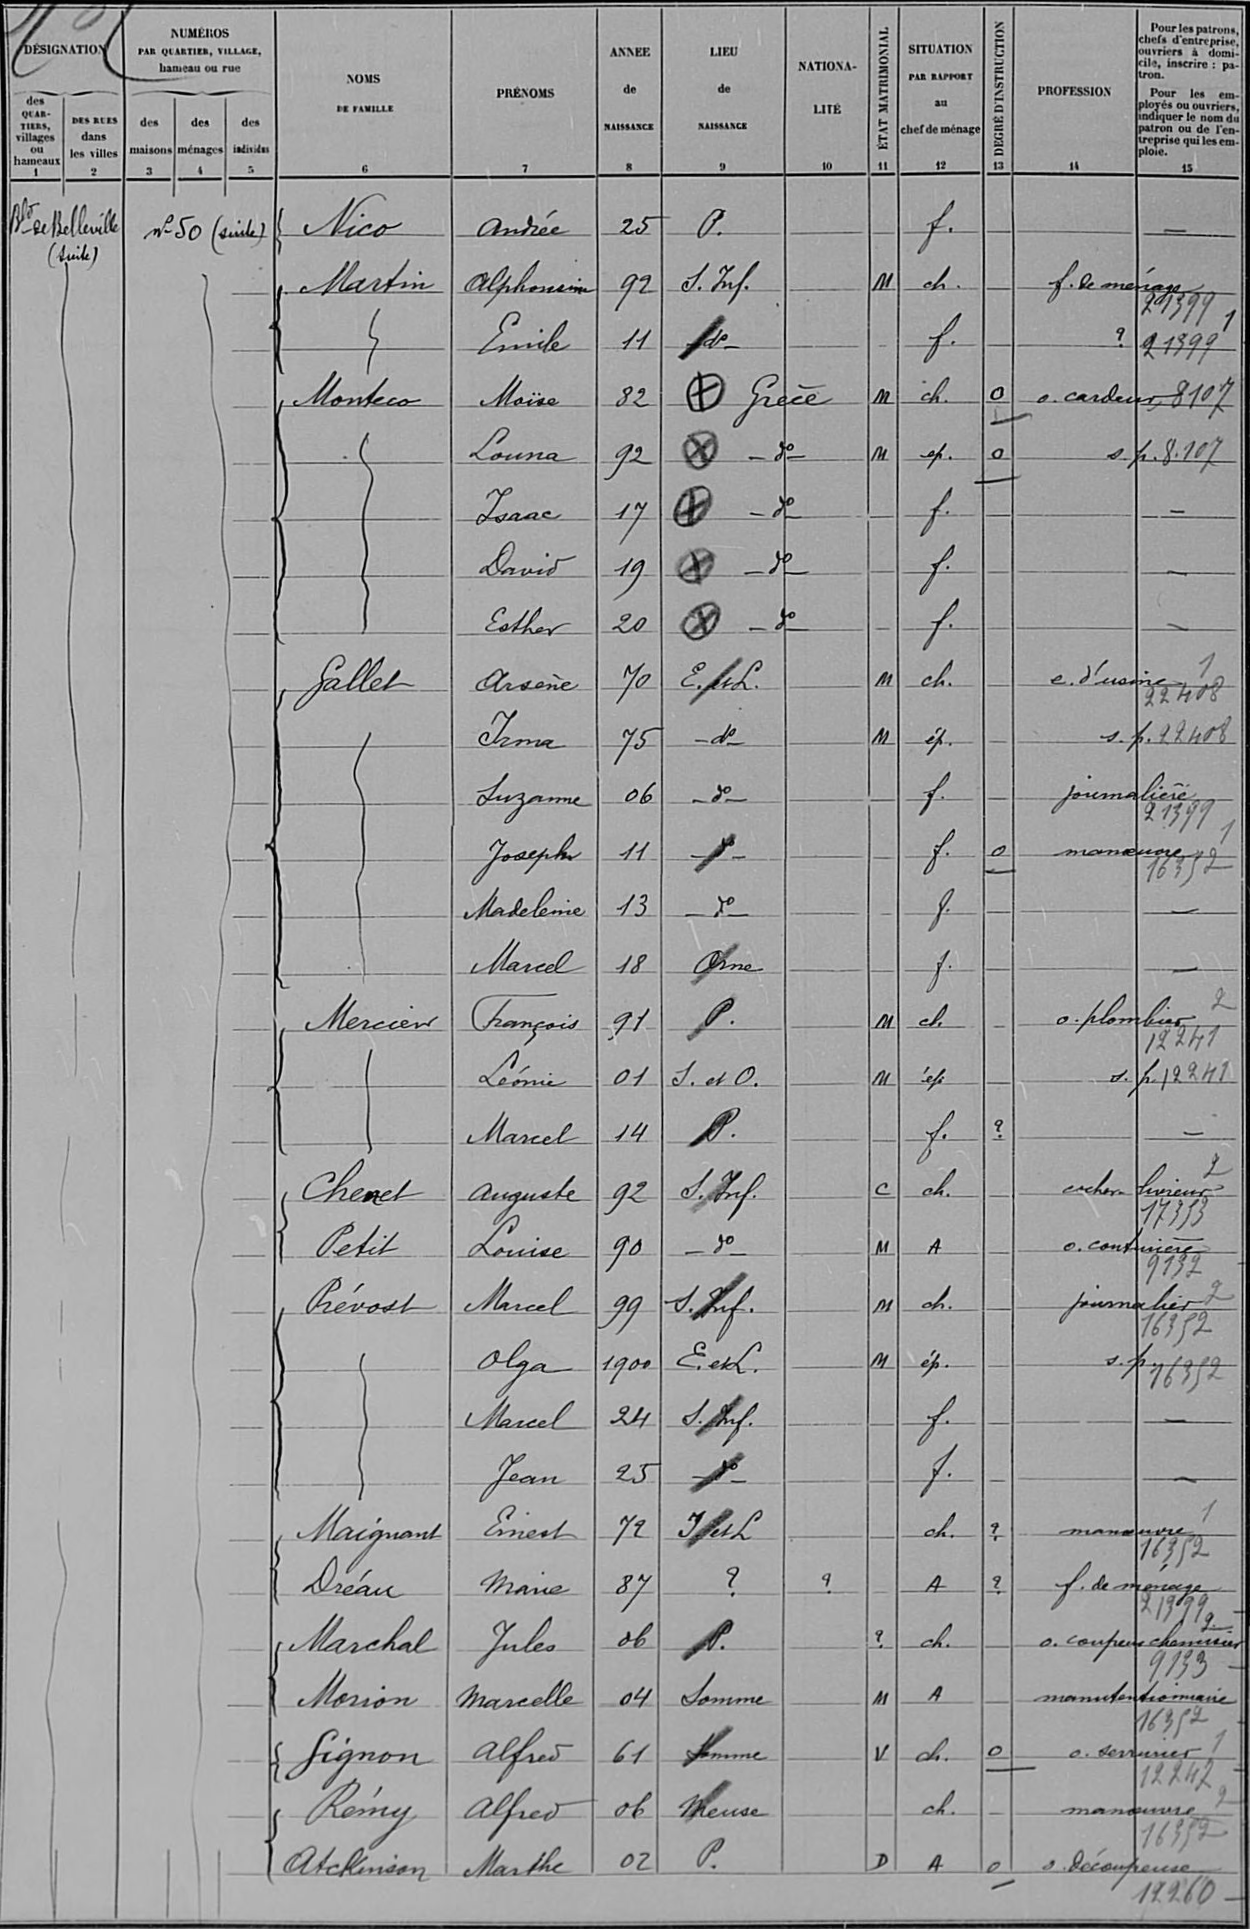Nico/Andrée/25/P /¤/¤/f /¤/¤/¤/¤
Martin/Alphonsine/92/S Inf /¤/M/ch /¤/f de ménage/29399
¤/Emile/11/d°/¤/¤/f /¤/¤/213991
Monteco/Moïse/82/Grèce/¤/M/ch /o/o cardeur/8107
¤/Louna/92/d°/¤/M/ep /o/s p /8107
¤/Isaac/17/d°/¤/¤/f /¤/¤/¤/¤
¤/David/19/d°/¤/¤/f /¤/¤/¤/¤
¤/Esther/20/d°/¤/¤/f /¤/¤/¤/¤
Gallet/Arsène/70/E et L /¤/M/ch /¤/e d'usine/22408 1
¤/Irma/75/d°/¤/M/ép /¤/s p /22408
¤/Suzanne/06/d°/¤/¤/f /¤/journalière/21399
¤/Joseph/11/d°/¤/¤/f /o/manoeuvre/¤
¤/Madeleine/13/d°/¤/¤/f /¤/¤/¤/¤
¤/Marcel/18/Orne/¤/¤/f /¤/¤/¤/¤
Mercier/François/91/P /¤/M/ch /¤/o plombier/122412
¤/Léonie/01/S et O /¤/M/ép/¤/s p /12241
¤/Marcel/14/P /¤/¤/f /¤/¤/¤/¤
Chenet/Auguste/92/S Inf /¤/c/ch /¤/cocher livreur/¤
Petit/Louise/90/d°/¤/M/A/¤/o couturière/¤
Prévost/Marcel/99/S Inf /¤/M/ch /¤/journalier/¤
¤/Olga/1900/E et L /¤/M/ép /¤/s p /¤
¤/Marcel/24/S Inf /¤/¤/f /¤/¤/¤/¤
¤/Jean/25/d°/¤/¤/f /¤/¤/¤/¤
Maignant/Ernest/72/I et L/¤/¤/ch /¤/manoeuvre/¤
Dréau/Marie/87/¤/¤/¤/A/¤/f de ménage/¤
Marchal/Jules/06/P /¤/¤/ch /¤/o coupeur chemisier/¤
Morion/Marcelle/04/Somme/¤/M/A/¤/manutentionnaire/¤
Gignon/Alfred/61/Somme/¤/V/ch /O/o serrurier/¤
Rémy/Alfred/06/Meuse/¤/¤/ch /¤/manoeuvre/¤
Atckinson/Marthe/02/P /¤/D/A/O/o découpeuse/¤
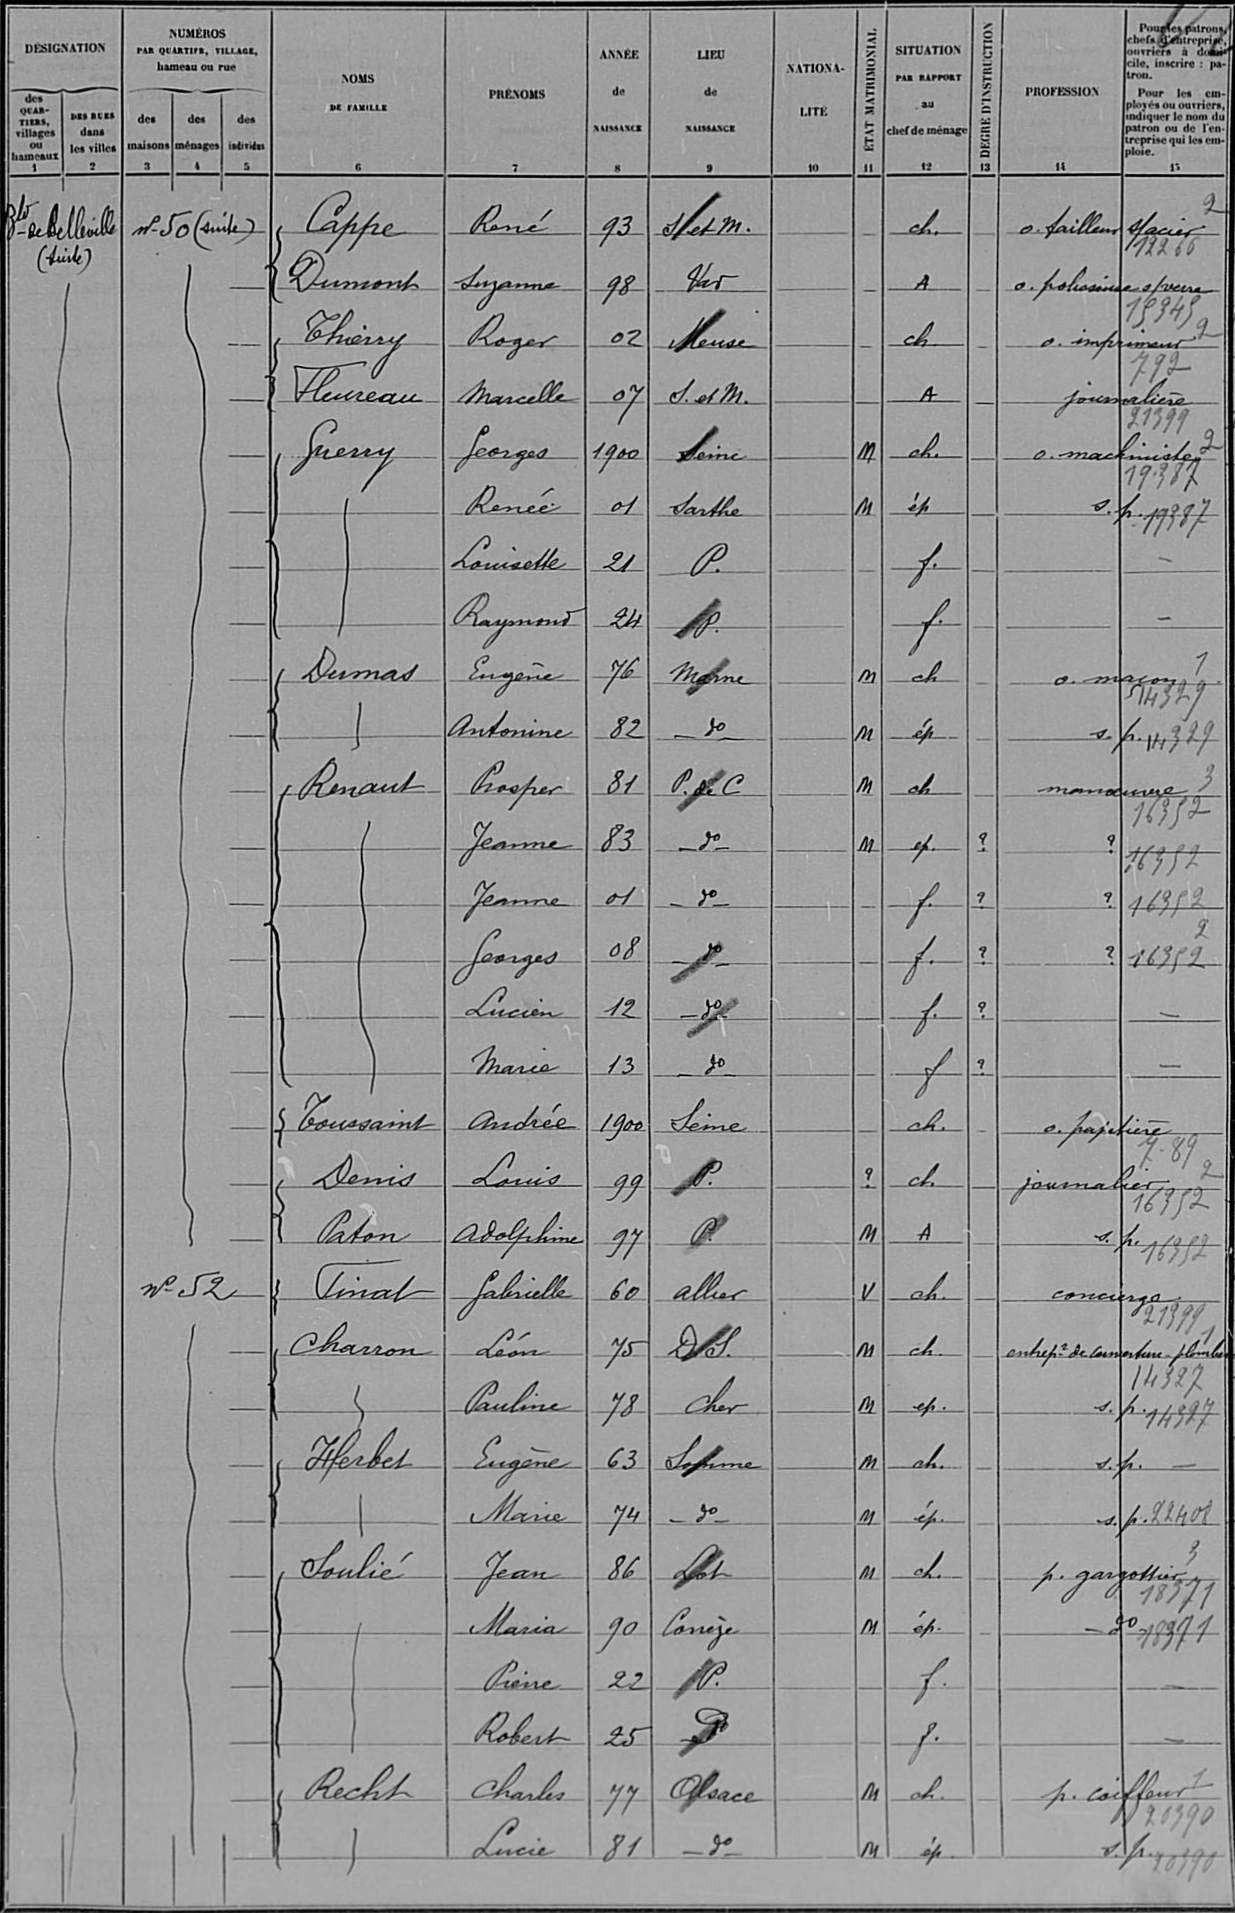Cappe/René/93/S et M /¤/¤/ch /¤/o tailleur s- acier/¤
Dumont/Suzanne/98/Var/¤/¤/A/¤/o polisseuse s- verre/¤
Thierry/Roger/02/Meuse/¤/¤/ch/¤/o imprimeur/¤
Fleureau/Marcelle/07/S et M /¤/¤/A/¤/journalière/¤
Guerry/Georges/1900/Seine/¤/M/ch /¤/o machiniste/¤
¤/Renée/01/Sarthe/¤/M/ép/¤/s p /19387
¤/Louisette/21/P /¤/¤/f /¤/¤/¤/¤
¤/Raymond/24/P /¤/¤/f /¤/¤/¤
Dumas/Eugène/76/Marne/¤/M/ch/¤/o maçon/¤
¤/Antonine/82/d°/¤/M/ép/¤/s p /14329
Renaut/Prosper/81/P de C/¤/M/ch/¤/manoeuvre/¤
¤/Jeanne/83/d°/¤/M/ep /¤/¤/16352
¤/Jeanne/01/d°/¤/¤/f /¤/¤/16352
¤/Georges/08/d°/¤/¤/f /¤/¤/¤/¤
¤/Lucien/12/d°/¤/¤/f /¤/¤/¤/¤
¤/Marie/13/d°/¤/¤/f /¤/¤/¤/¤
Toussaint/Andrée/1900/Seine/¤/¤/ch /¤/o papetière/7 89
Denis/Louis/99/P /¤/¤/ch/¤/journalier/¤
Paton/Adolphine/97/P /¤/M/A/¤/s p /16352
Vinat/Gabrielle/60/Allier/¤/V/ch /¤/concierge/¤
Charron/Léon/75/D S /¤/M/ch /¤/entrepr de couverture plomberie/¤
¤/Pauline/78/Cher/¤/M/ep /¤/s p /14327
Herbet/Eugène/63/Somme/¤/M/ch /¤/s p /¤
¤/Marie/74/d°/¤/M/ép /¤/s p /22408
Soulié/Jean/86/Lot/¤/M/ch /¤/p gargottier/¤
¤/Maria/90/Corrèze/¤/M/ép /¤/d°/18371
¤/Pierre/22/P /¤/¤/f /¤/¤/¤/¤
¤/Robert/25/P/¤/¤/f /¤/¤/¤/¤
Recht/Charles/77/Alsace/¤/M/ch /¤/p coiffeur/¤
¤/Lucie/81/d°/¤/M/ép /¤/s p /¤
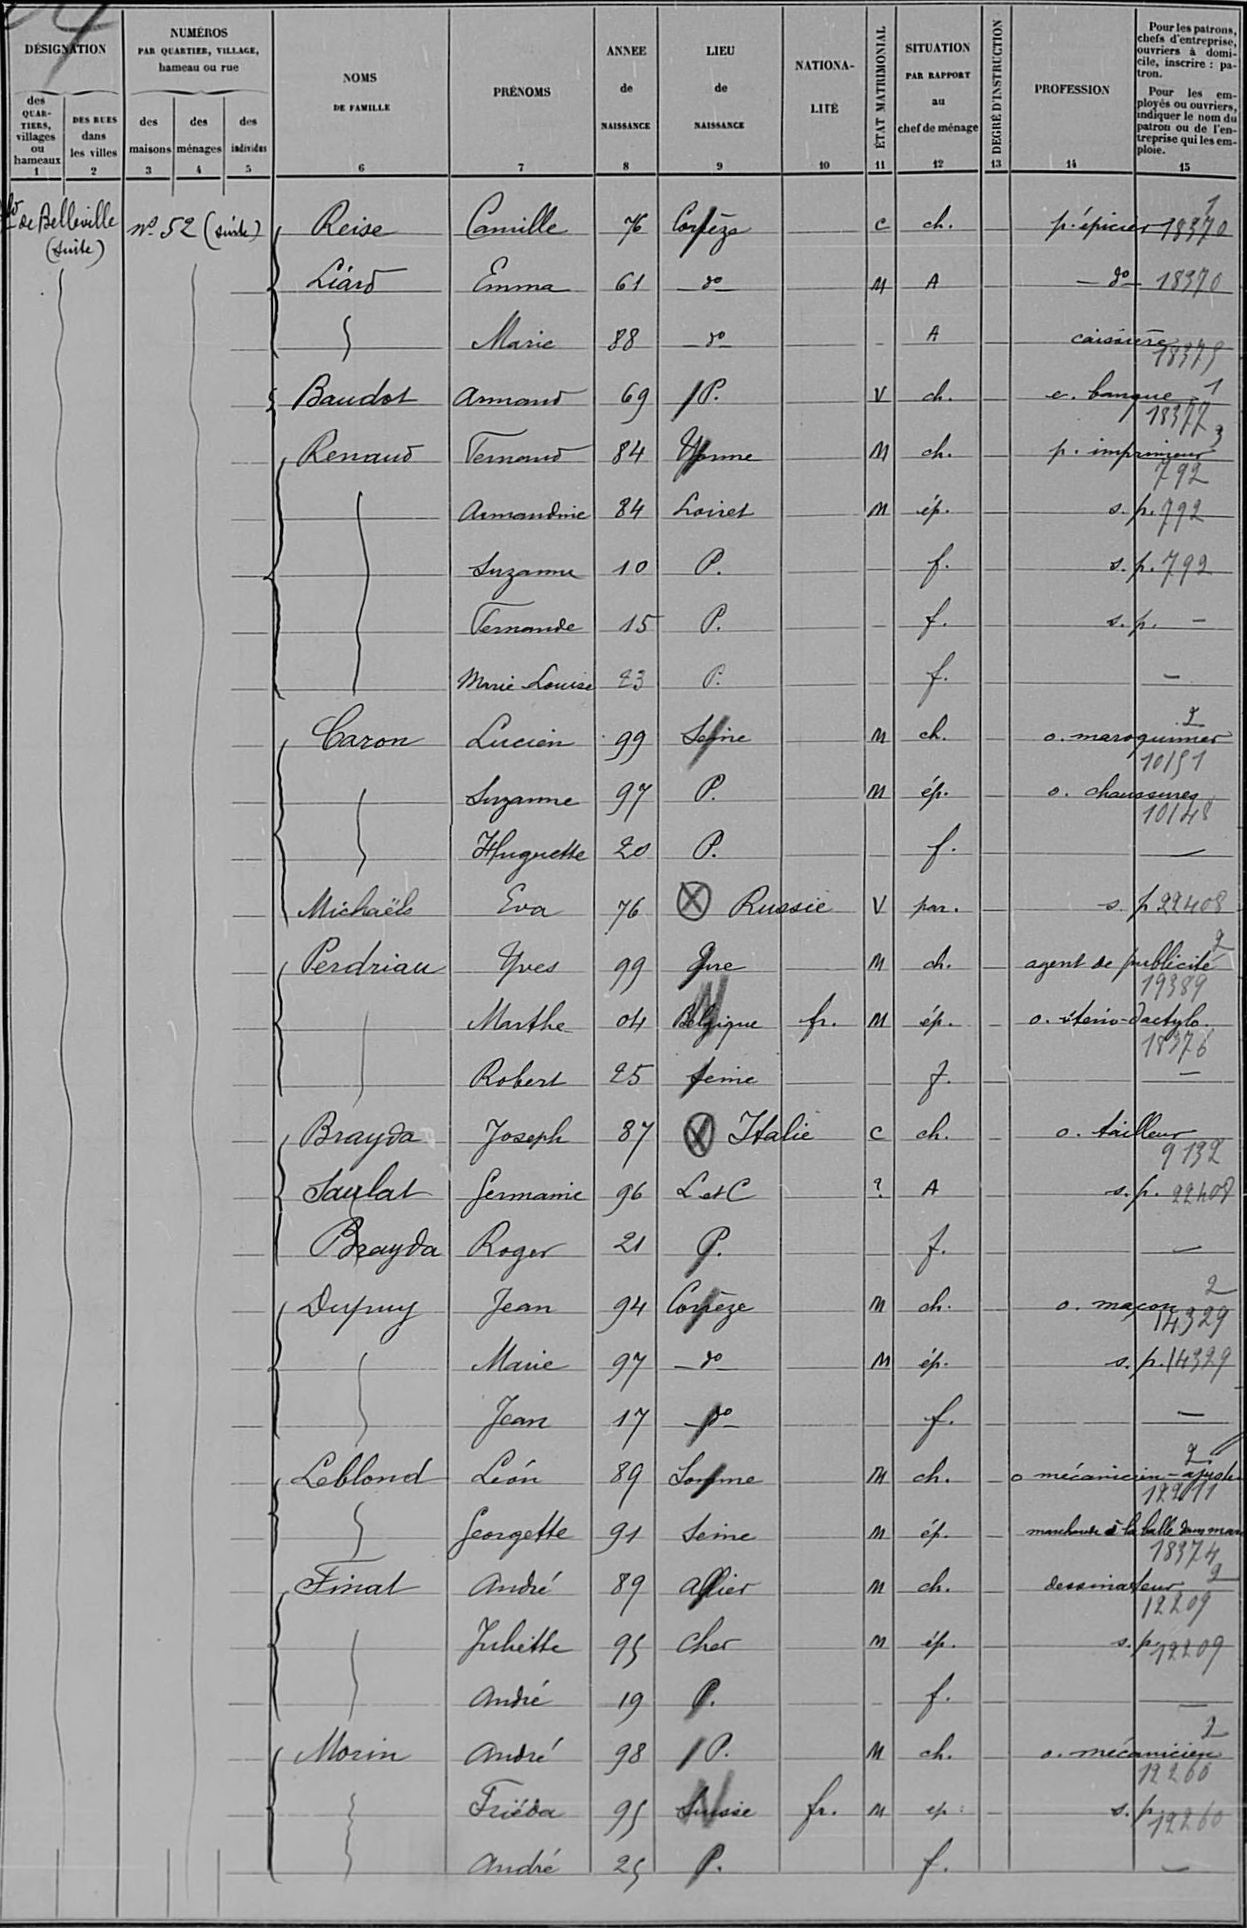Reise/Camille/76/Corrèze/¤/c/ch /¤/p épicier/18370
Liard/Emma/61/d°/¤/M/A/¤/d°/18370
¤/Marie/88/d°/¤/¤/A/¤/caissière/¤
Baudot/Armand/69/P /¤/V/ch /¤/e banque/183721
Renaud/Fernand/84/Yonne/¤/M/ch /¤/p imprimeur/¤
¤/Amandine/84/Loiret/¤/M/ép /¤/s p /792
¤/Suzanne/10/P /¤/¤/f /¤/s p /792
¤/Fernande/15/P /¤/¤/f /¤/s p /¤/¤
¤/Marie-Louise/23/P /¤/¤/f /¤/¤/¤/¤
Caron/Lucien/99/Seine/¤/M/ch /¤/o maroquinier/¤
¤/Suzanne/97/P /¤/M/ép /¤/o chaussures/¤
¤/Huguette/20/P /¤/¤/f /¤/¤/¤/¤
Michaëls/Eva/76/Russie/¤/V/par /¤/s p/22408
Perdriau/Yves/99/Eure/¤/M/ch /¤/agent de publicité/¤
¤/Marthe/04/Belgique/fr /M/ép /¤/o sténo dactylo/¤
¤/Robert/25/Seine/¤/¤/f /¤/¤/¤/¤
Brayva/Joseph/87/Italie/¤/c/ch /¤/o tailleur/¤
Saylat/Germaine/96/L et C/¤/¤/A/¤/s p /22408
Breyva/Roger/21/P /¤/¤/f /¤/¤/¤/¤
Dupuy/Jean/94/Corrèze/¤/M/ch /¤/o maçon/¤
¤/marie/97/d°/¤/M/ép /¤/s p /14329
¤/Jean/17/d°/¤/¤/f /¤/¤/¤/¤
Leblond/Léon/89/Somme/¤/M/ch /¤/o mécanicien-ajusteur/¤
¤/Georgette/91/Seine/¤/M/ép /¤/marchande à la belle /¤
Finat/André/89/Allier/¤/M/ch /¤/dessinateur/¤
¤/Juliette/95/Cher/¤/M/ép /¤/s p /12209
¤/André/19/P /¤/¤/f /¤/¤/¤/¤
Morin/André/98/P /¤/M/ch /¤/o mécanicien/¤
¤/Frieda/95/Suisse/fr /M/ep/¤/s p /12260
¤/André/25/P /¤/¤/f /¤/¤/¤